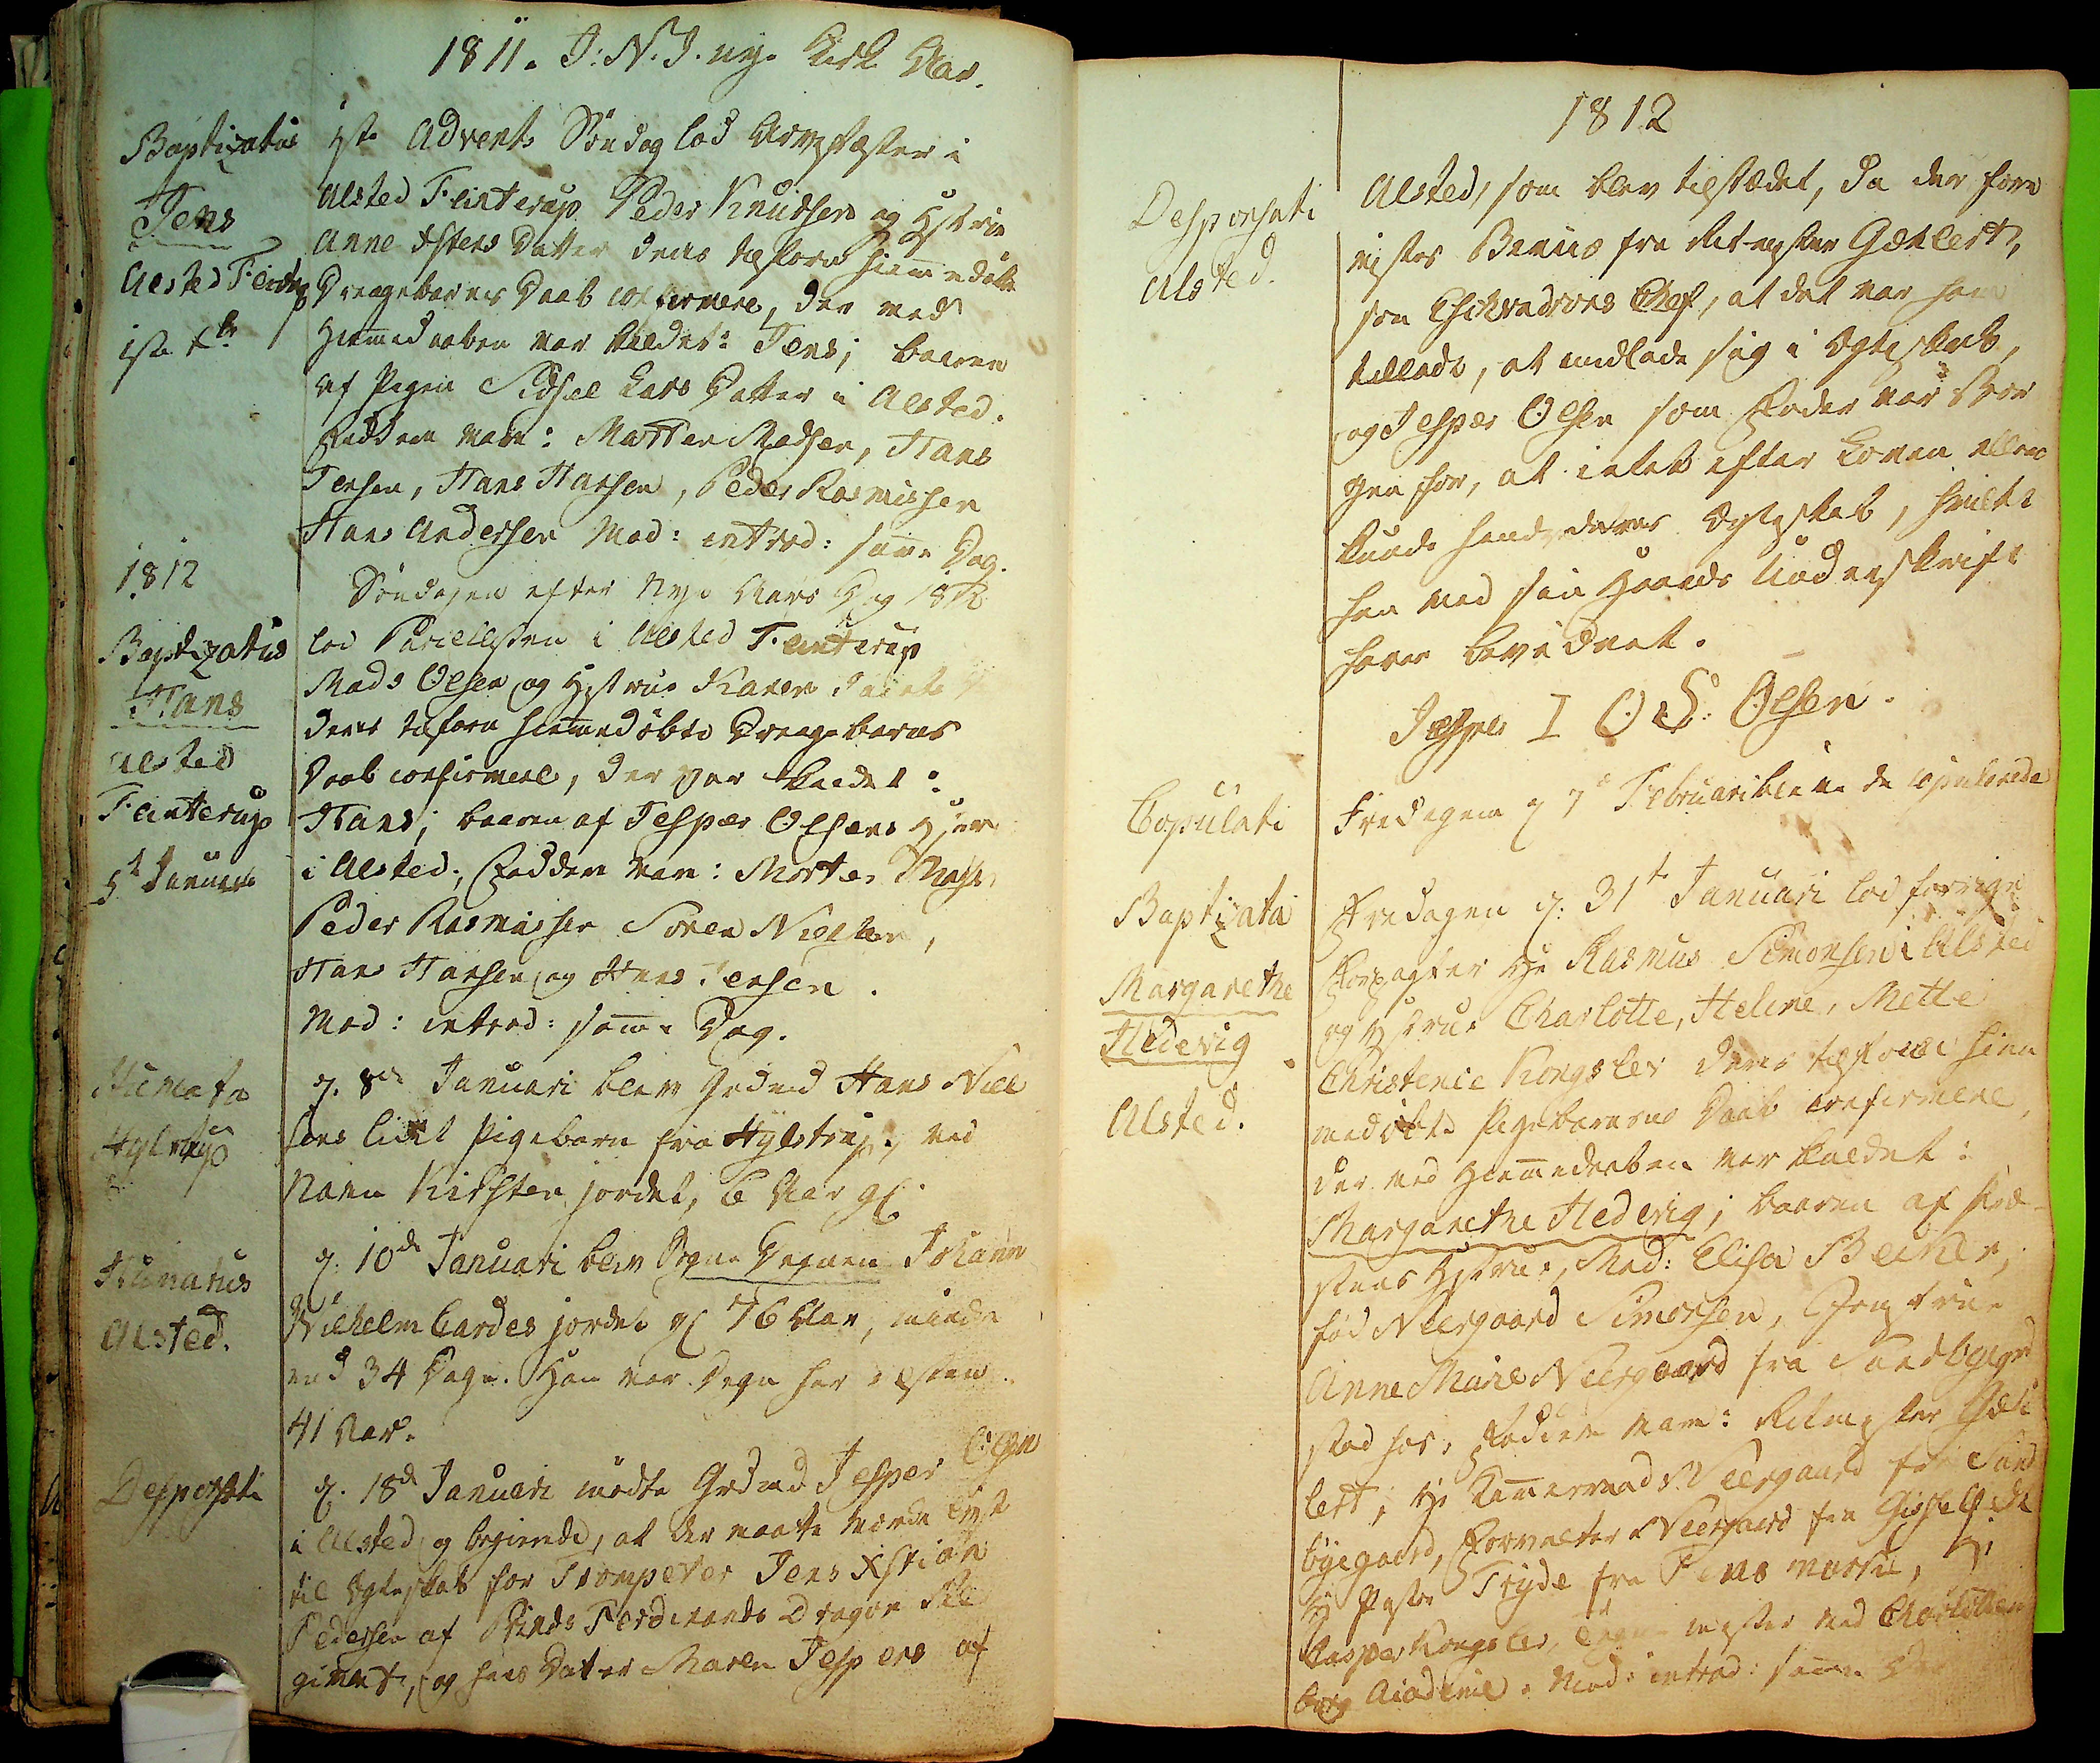Baptizatus
Jens
Alsted Flinterup
1ste Xbr
1812
Baptizatus
Hans
Alsted
Flinterup
5te Januar
Humata
Hylstrup
Humatus
Alsted.
Despensati
1811. J: N. J. nye  Kirke Aar.
1ste Advents Søndag lod Arvefæster i
Alsted Flinterup Peder Knudsen og Hstrue
Anne Xstens Datter deres tilforn hiemmedøbte
Drengebarns Daab confirmere, der ved
Hiemmedaaben var kaldet: Jens, baaren
af Pigen Sidsel Lars Datter i Alsted.
Faddere vare: Morten Madsen, Hans
Jensen, Hans Hansen, Peder Rasmussen
Hans Andersen Mod: introd: samme Dag.
Søndagen efter Nye Aars HDag 1812
lod Parcellisten i Alsted Flinterup
Mads Olsen og Hstrue Karen Jacobs
deres tilforn hiemmedøbte Drengebarns
Daab confirmere, der var kaldet:
Hans, baaren af Jesper Olsens Hstr
i Alæsted; Faddere var: Morten Ma##
Peder Rasmussen Søren Nielsen
Hans Hansen og Hans Jensen
Mod: introd: samme Dag.
d. 8de Januari blev Grdmd Hans Niel
sens lidet Pigebarn fra Hylstrup, ved
navn Kirsten jordet 6 Aar gl.
d: 10de Januari blev Sogne Degnen Johann
Wilhelm Cardes jordet gl 76 Aar, mindre
end 34 Dage. Han var Degn her i Alsted
41 Aar.
d: 18de Januari mødte Grdmd Jesper Olsen
i Alsted, og begierede, at der maatte vorde lyst
til Ægteskab for Trompeter Jens Xstian
Pedersen af ##ds Ferdinants Dragon Re
giment, og hans Datter Maren Jespers af
Desponsati
Elsted.
Copulati
Baptizata
Margarethe
Hedevig
Alsted.
1812
Alsted, som blev tilstædet, da der fore
vistes Bevis fra Ritmester G##lert,
som Esckradrons Chef, at det var ham
tilladt, at indlade sig i Ægteskab,
og Jesper Olsen som Fader var Bor¬
gen for, at intett efter Loven ellers
kunde hindre deres Ægteskab, hvilketHans
han ved sina Haandsns Underskrift
have bevidnet.
Jesper I O S Olsen
Fredagen d 7 Februari bleve de copulerede
Fredagen d: 31te Januari lod forrige
Forpagter Hr Rasmus Simonsens i Alsted
og Hstrue Charlotte, Helene, Mette
Christence Kongslev deres tilfolde hiem¬
medøbte Pigebarns Daab confirmere
der ved Hiemmedaaben var kaldet:
Margarethe Hedevig; baaren af Præ¬
stens Hstrue, Mad: Elise Becker,
fød Neergaard Simonsen, Jomfrue
Anne Marie Nergaard fra Sandbyegrd
stod hos, Faddere vare: Ritmester G##
lert, Hr Kammerraad Neergaard fra Sand
byegaard, Forvalter Neergaard fra Gisselfelt
Hr Pastor Tryde fra Fensmark##,
Casper Kongslev, T## ##sted ved Char##
borg Aiodenil. Mod: introd: samme Dag
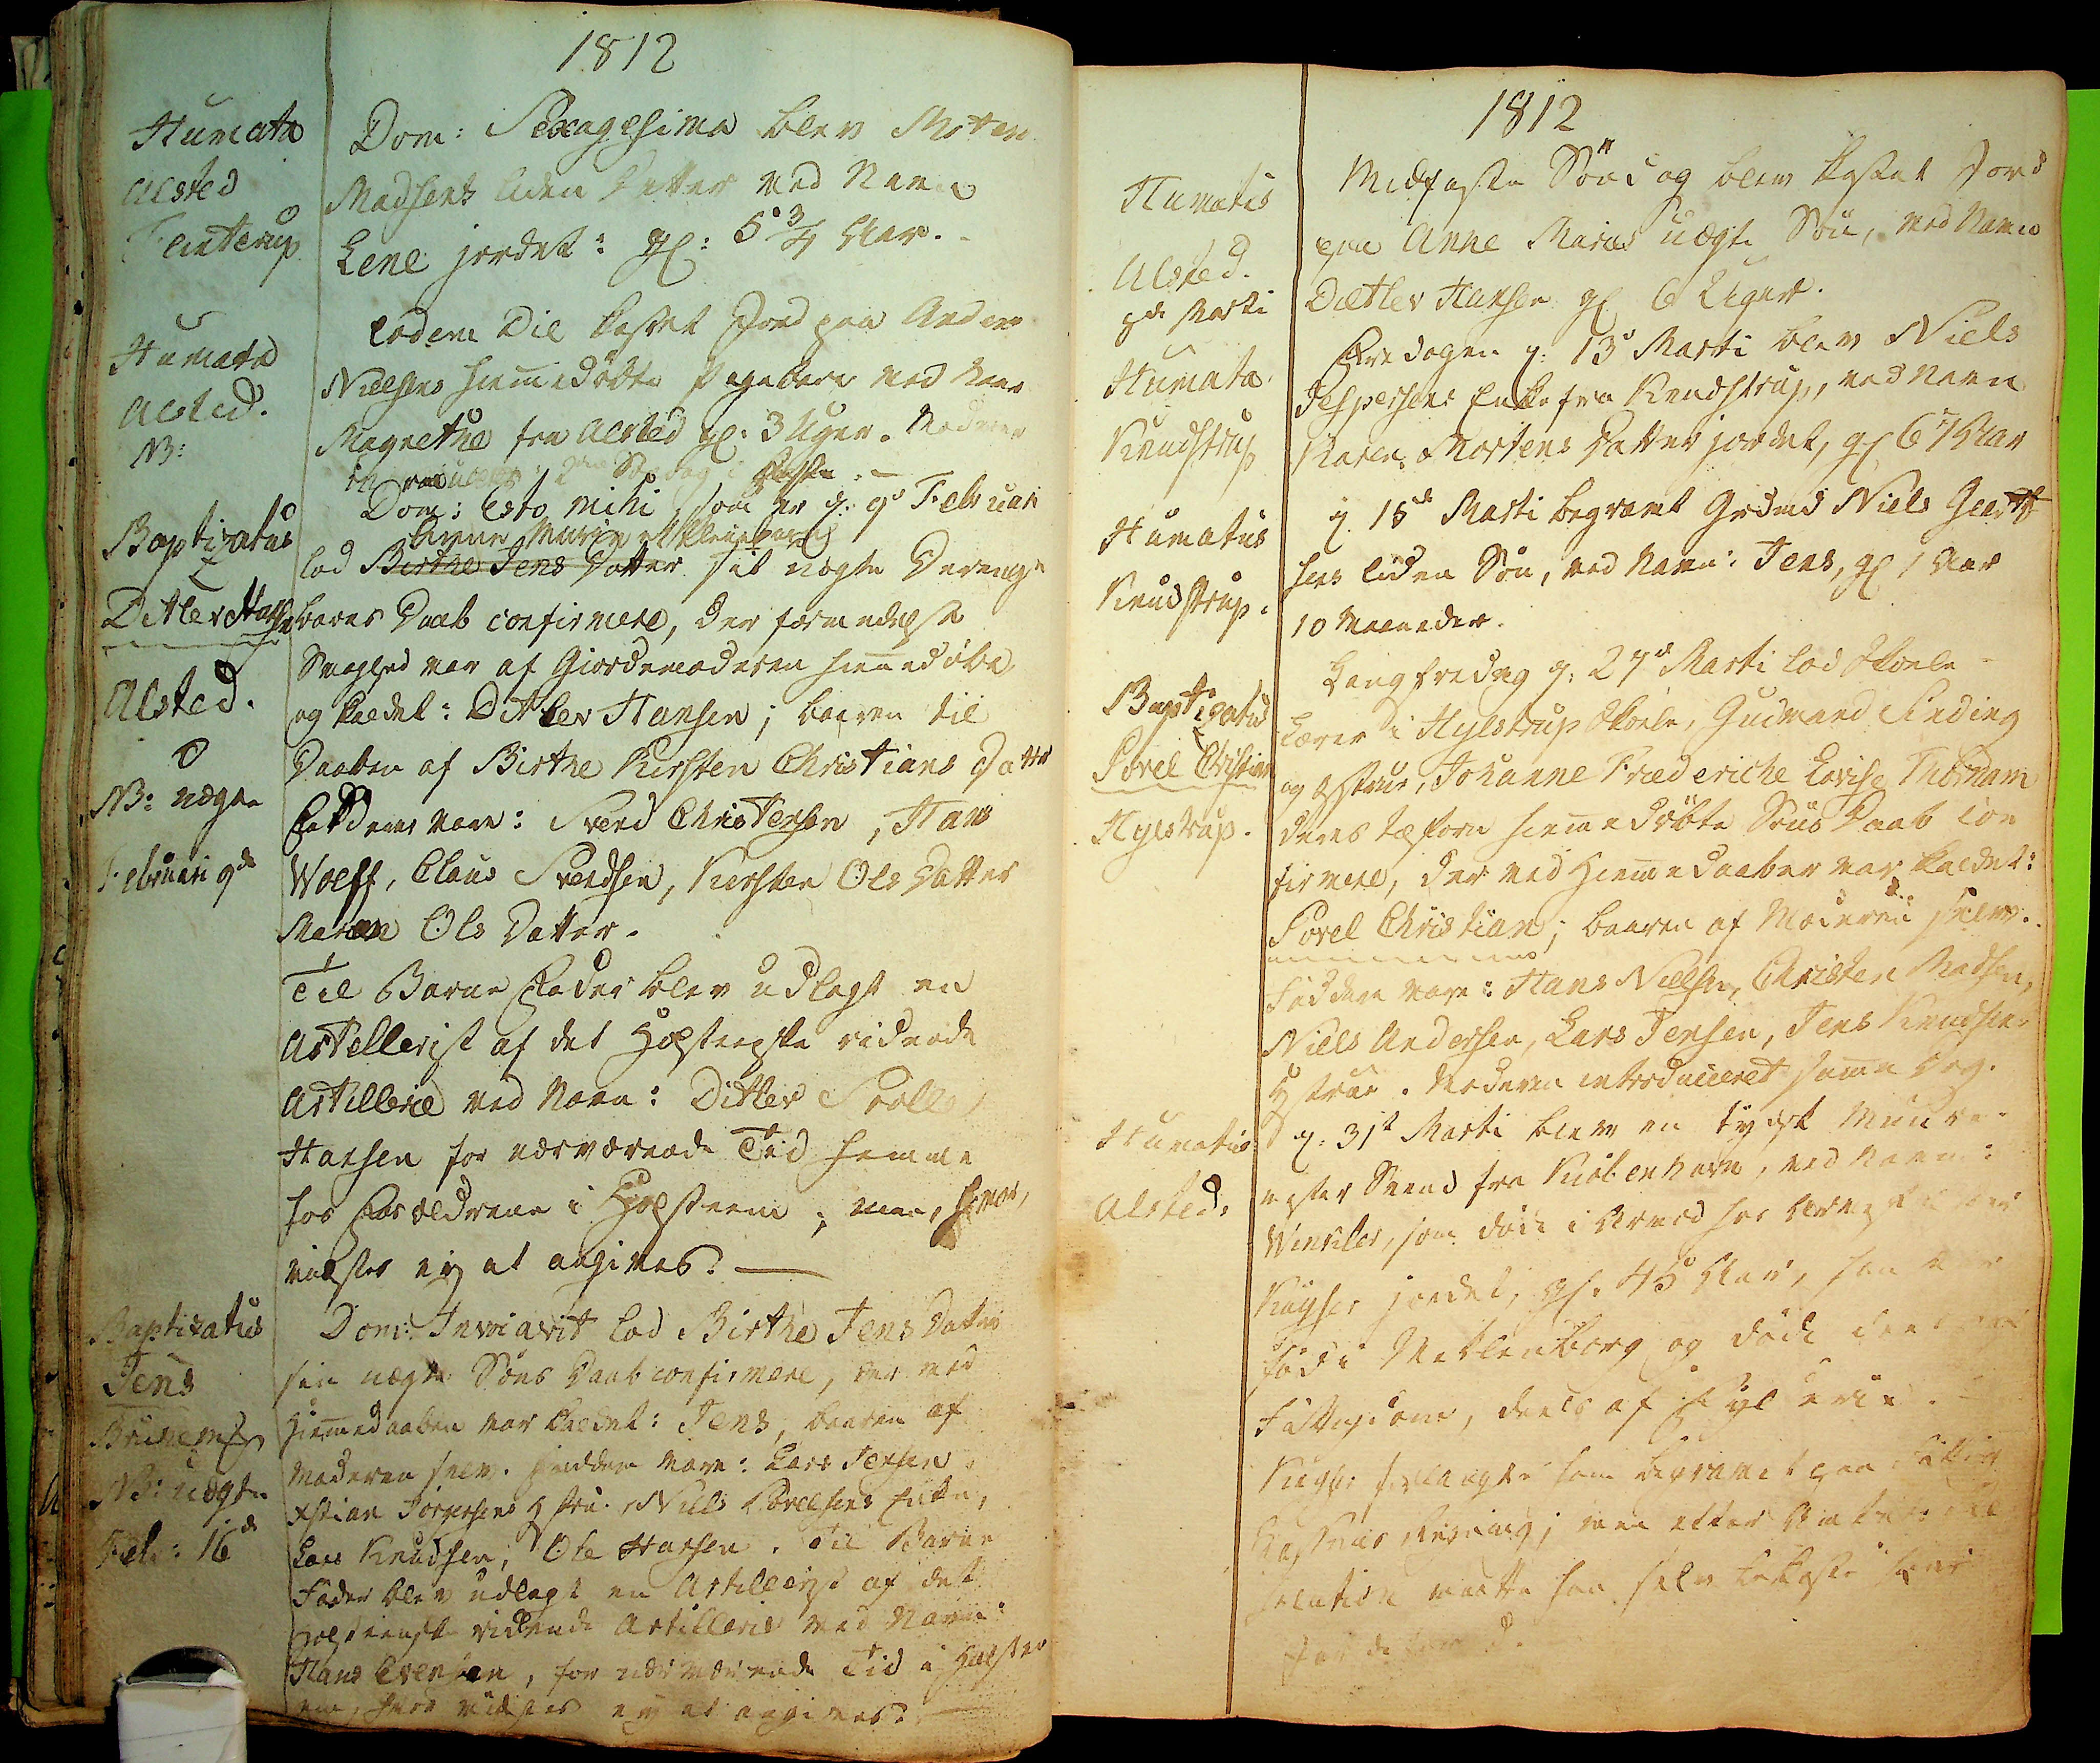Humata
Alsted
Flinterup
Humata
Alsted.
NB:
Baptizatus
Ditlev Hansen
Alsted.
NB: uægte
Februari 9de
Baptizatus
Jens
Brunem##
NB: uægte
Febr: 16de
1812
Dom: Sexagesima blev Morten
Madsens liden Datter ved Navn
Lene jordet: gl: 5¾ Aar.
Eodem Die kastet Jord paa Anders
Nielsens hiemmedøbter Pigebarn ved Navn
Magrethe fra Alsted gl: 3 Uger. Moderen
introduceret 2den Søndag i Fasten
Dom: Esto mihi som er d: 9de Februari
Anne Marie af Flinterup
lod Birthe Jens Datter sit uægte Drenge
barns Daab Confirmere, der formedelst
Svaghed var af Giordemoderen hiemmedøbt
og kaldet: Ditlev Hansen, baaren til
Daaben af Birthe Kirsten Christians Datter
Fadderne vare: Svend Christensøn, Hans
Wolff, Claus Svendsen, Kirsten Ols Datter
Maren Ols Datter.
Til Barnefader blev udlagt en
Artillerist af det Holstenske ridnede
tArtillerie ved Nafn: Ditlev S##olle
Hansen for nærværende Tid Hemme
hos Forældrene i Holsteen; men hvor
vistes ey at angives.
Dom: Invocavit lod Birthe Jens Datter
sin uægte Søns Daab confirmere, der ved
Hiemmedaaben var kaldet: Jens, baaren af
Moderen selv. Faddere vare: Lars Jensen
Xstian Jørgensens Hstru, Niels Povelsens Enke
Lars Knudsen, Ole Hansen, Til Barne
Fader blev udlagt en Artillerist af det
Holstenske ridende Artillerie ved Navn:
Hans Evensen, for nærværende Tid i Holsteen
men hvor vidstes ey at angives.
Humatus
Alsled.
8de Marti
Humata
Knudstrup
Hamatus
Knudstrup.
Baptizatus
Povel Christian
Hylstrup.
Humatus
Alsted.
1812
Midfaste Søndag blev kastet Jord
paa Anne Maries uægte Søn ved Navn
Dætlev Hansen gl 6 Uger.
Fredagen d: 13de Marti blev Niels
Jespersens Enke fra Knudstrup, ved Navn
Karen Mortens Datter jordet gl 67 Aar
d: 15de Marti begravet Grdmd Niels Geert
sens liden Søn, ved navn: Jens, gl 1 Aar
10 Maaneder.
Langfredag d: 27 Marti lod Skole¬
Lærer i Hulstrup Skoele, Gudmand Sinding
og Hstrue, Johanne Frederiche Lovise Thoman
deres tilforn hiemmedøbte Søns Daab con
firmere, der ved Hiemedaaben var kaldet:
Povel Christian, baaren af Moderen selv.
Faddere vare: Hans Nielsen, Christen Madsen
Niels Anderssen, Lars Jensen, Jens Knudsens
Hstrue. Moderen introduceret samme Dag.
d: 31te Marti blev en tydsk Muure
mester Svend fra Kiøbenhavn, ved Navn:
Winkler, som døde i Armod hos Arvefæster 
Kayser jordet gl: 45 Aar, han var
fød i Meklenborg og døde ##
Fattigdom, dels af ##
Kayser forlangte ham begravet paa Fattig
## Ringning; ## efter Amt## Re
solutiom maatte han selv bekoste ##
## ##